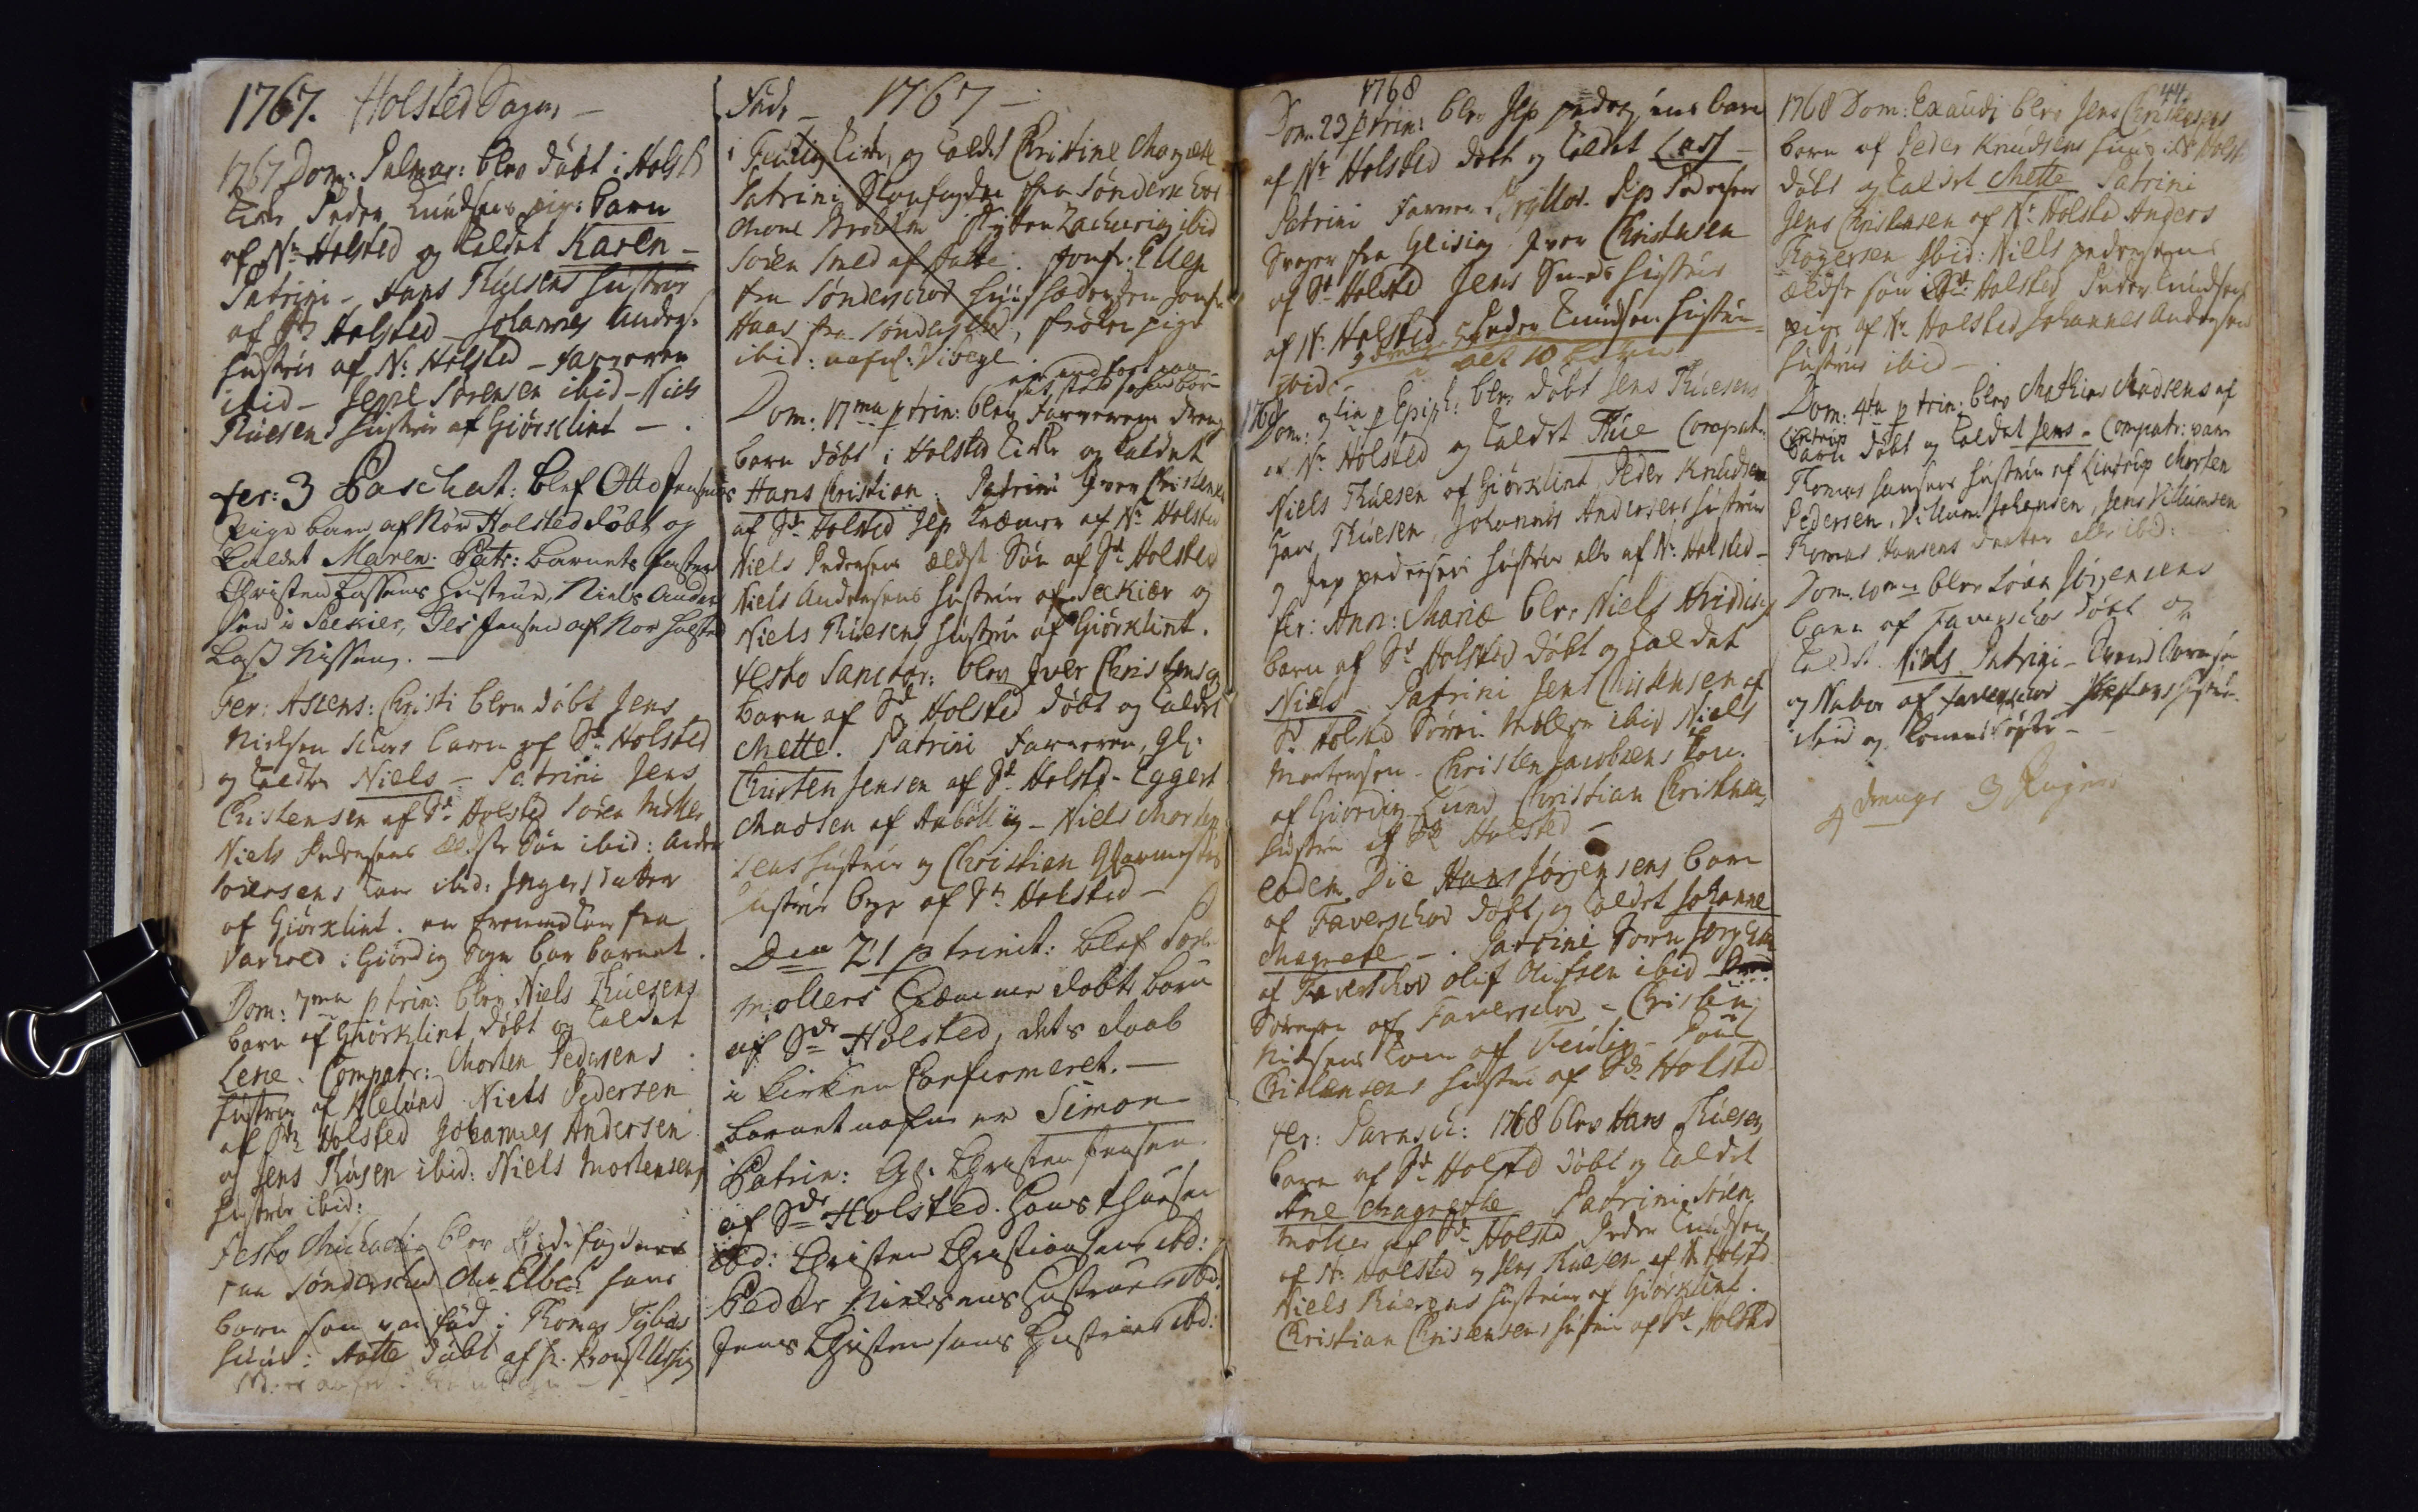1767. Holsted Sogns
1767 Dom: Palmar: blev døbt i Holsted
Kirke Peder Knudsens pige barn
af Nr Holsted og kaldet Karen -
Patrini - Hans Thuesens Hustrue
af Sdr Holsted Johannes Anders:
Hustru af N: Holsted - Farveren
ibid - Jeppe Sørrensen ibid - Niels
Tuesens Hustru af Giørklint -
Fer: 3 Paschat: blef Otto Jensens
Pige barn af Nør Holsted døbt og
kaldet Maren. Patr: Barnets Faster
Christen Lassens Hustrue, Niels Ander¬
sen i Seekier, Jes Jensen af Nor Holsted
Lass Nissen.
Fer: Ascens: Christi blev døbt Jens
Nielsen Sc##s barn af Sdr Holsted
og kaldet Niels - Patrini Jens
Christensen af Sdr Holsted Søren Møller
Niels Pedersens ældste Søn ibid: Ander
Sørensens Kone ibid. Ingers datter
af Giørklint. en fremmed Kone fra
Varhold i Giørding Sogn bar barnet.
Dom: 7ma p Trin: blev Niels Thuesens
Barn af Giørkhlint døbt og kaldet
Lene Compatr: Morten Pedersens
Hustrue af Klelund Niels Pedersen
af Sdr Holsted Johannes Andersen
og Jens Thusen ibid: Niels Mortensens
Hustrue ibid:
Festo Michaelis blev Ridefogdens
paa JSønderschov ## Elbes hans
barn som var fød i Thomas Pybas
huus i MAatte døbt af Hr. Proust Ussig
M##
1767
Føde -
i Feuling Kirke og kaldet Christine Magrete
Patrini Skoufogden fra Sønderschov
## ##roholm skytten Zacharis ibid
Søren Smed af Aatte. Jomfr Ellen
fra Sønderschov Huusholderen Jomfr
Haar fra Sønderschov, Frøkenepige
ibid: nafnl: Vibegl
## indfort paa
##et stod ## bør
Dom: 17ma p Trin: blev Faverens drenge
barn døbt i Holsted Kirke og kaldet
Hans Christian: Patrini Iver Christensen
af Sdr Holsted Jep Kræmer af Nr Holsted
Niels Pedersens ældste Søn af Sdr Holsted
Niels Andersens Hustrue af Seekier og
Niels Thuesens Hustrue af Giørklint.
Festo Sanctor: blev Iver Christensens
barn af Sdr Holsted døbt og kaldet
Mette. Patrini Farveren, gl:
Christen Jensen af Sdr Holsted Eggert
Madsen af Aabølling - Niels Morten¬
sens Hustrue og Christian Glarmesters
Hustrue bege af Sdr Holsted -
Dca 21 p Trinit: blef Søren
Møllers Hiæmme døbte barn
af Sdr Holsted, dets Daab
i Kirken Confirmeret. -
Barnet nafn er Simon
Patrin: Gl: Christen Jensen
af Sdr Holsted, Hans Thuesen
ibd: Christen Christiansens ibd:
Peder Nielsens Hustrue ibd:
Jens Christensens Hustrue ibd:
1768
Dom: 23 p Trin: blev Jep pedersens barn
af Nr Holsted det og kaldet Lass
Patrini Farver Bryllov. ##p Pedersen
Svoger fra Gliding, Iver Christensen
af Sdr Holsted, Jens Smeds Hustrue
af Nr Holsted Peder Knudsens Hustru
ibid.
5 drenge 5 piger
i alt 10 Børn.
1768
Dom: 3tia p Epiph: blev døbt Jens Thuesens
af Nr Holsted og kaldet Thue Compatr:
Niels Thuesen af Giørklint, Peder Knudsen
Hans Thuesens, Johannes Andersens Hustrue
og Jep pedersens Hustrue alle af Nr Holsted
Fer: Ann: Mariæ blev Niels Hviddings
Barn af Sdr Holsted døbt og kaldet
Niels - Patrini Jens Christensen af
Sdr Holsted Søren Møller ibid Niels
Mortensen - Christen Jacobsens Kon.
af Giording Lund Christian Christensens
Hustru af Sdr Holsted -
eodem Die Hans Jørgensens barn
af Faverschov døbt, og kaldet Johanne
Magrete Patrini Iver Jorgensen
af Faverschov Oluf Nissen ibid
Sørensen af Faverschov - Christen
Nilsens Kone af Feuling - Poul
Christensens Hustru af Sdr Holsted
Fer: Parasch: 1768 blev Hans Thuesens
barn af Sdr Holsted døbt og kaldet
Ane Magrethe Patrini Søren
Møller af Sdr Holsted, Peder Knudsen
af Nr Holsted og Jens Thuesen af Sdr Holsted
Niels Thuesens Hustrue af Giørklint
Christian Christensens Hustrue af Sdr Holsted
44.
1768 Dom: Exaudi blev Jens Christensens
Barn af Peder Knudsens Huus i Sdr Holsted
døbt og kaldet Mette Patrini
Jens Christensen af Nr Holsted Anders
Thøgersen ibid: Niels pedersens
ældste søn Sdr Holsted Peder Knudsens
pige af Nr Holsted Johannes Andersens
Hustrue ibid -
Dom: 4ta p Trin: blev Mathias Madsens af
Lintrup
barn døbt og kaldet Jens Compatr: vare
Thomas Hansens Hustrue af Lintrup Morten
Pedersen, Villum Johansen, Jens Villumsen
Thomas Hansens Daatter alle ibid:
Dom 10ma blev ## Jørgensens
barn af Faverschov døbt og
kaldet Niels Patrini Svend Sorensen
og Naboe af Faverschov ## Hustru
ibid og Konens Søster -
4 Drenge 3 Piger
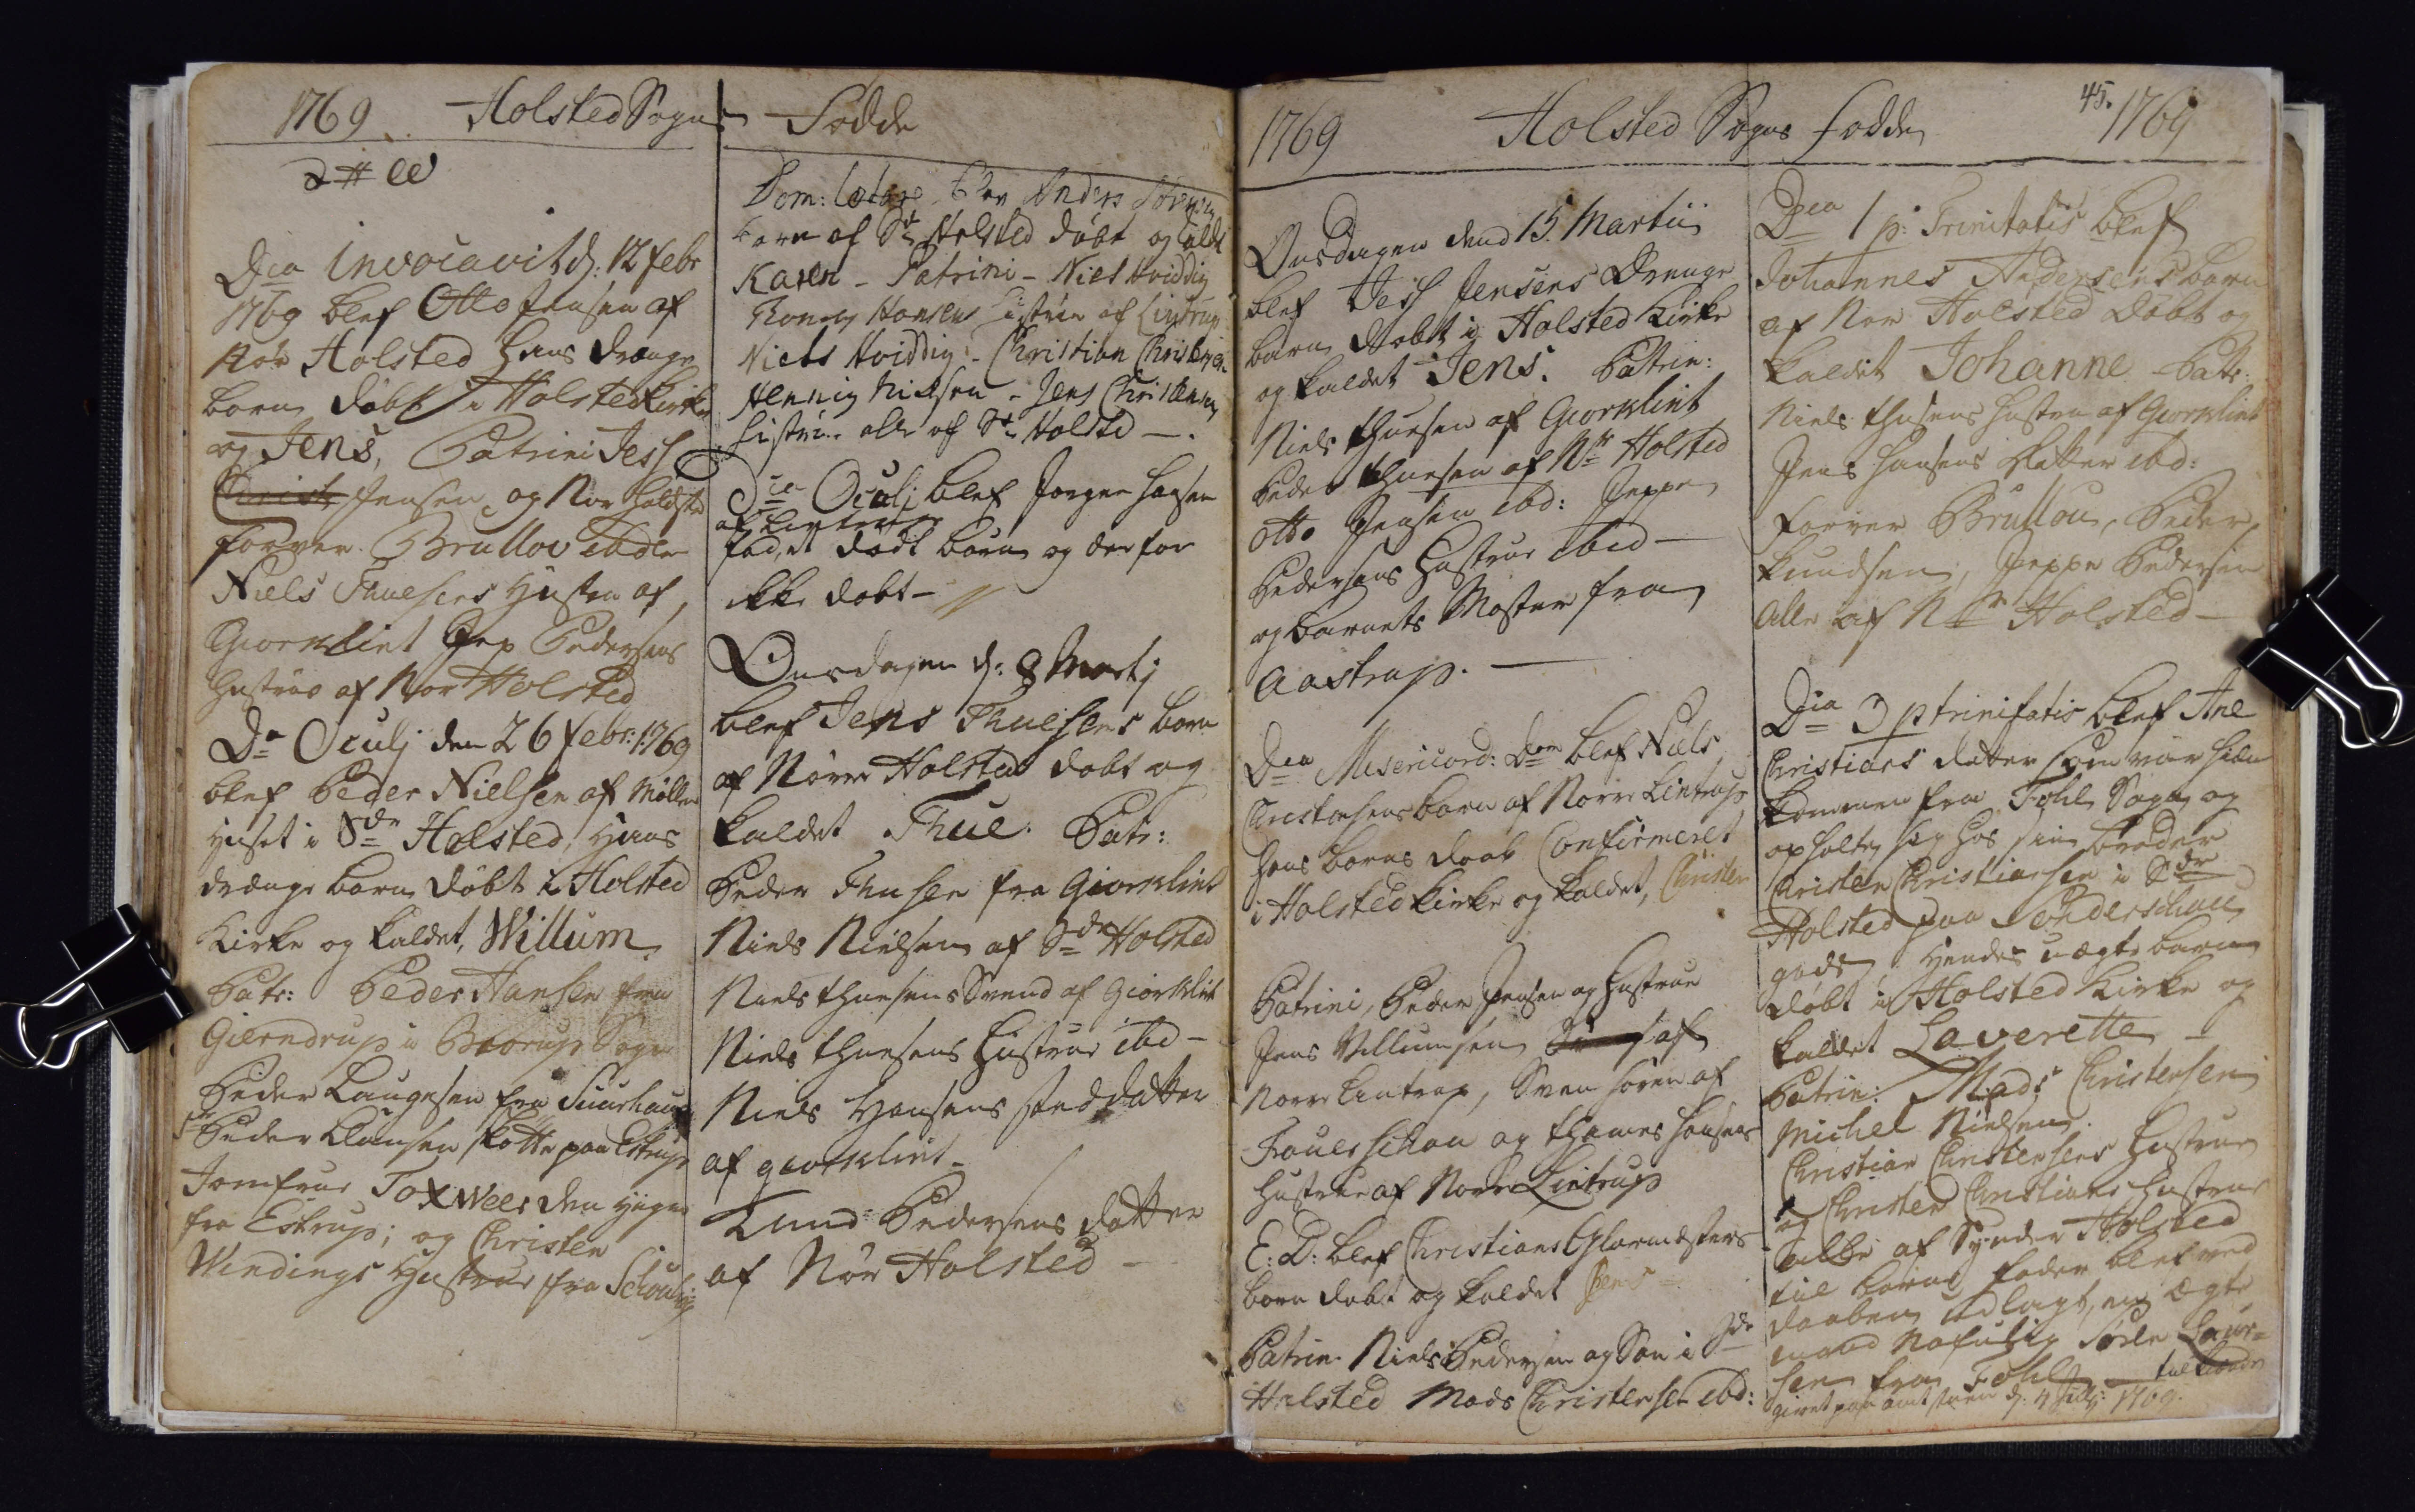Holted Sogns
1769
Dca Invocavit d: 12 Febr
1769 blef Otto Jensen af
Nør Holsted, Hans Drenge
barn døbt i Holsted Kirke
og Jens Patrini Jess
Christ Jensen og Nør Holsted
Forver Brullov ibidem
Niels Thuesens Hustru af
Giorklint Jep Pedersens
Hustrue af Nor Holsted
Dca Oculj den 26 Febr: 1769
blef Peder Nielsen af Mølle
Huuset i Sdr Holsted, Hans
drenge barn døbt i Holsted
Kirke og kaldet Willum.
Patr: Peder Hansen fra
Gierndrup i Brorup Sogn
Peder Laugesen fra Suurhauge
Peder Clausen Skøtte paa Estrup
Jomfrue Toxweer den yngre
fra Estrup; og Christen
Windings Hustrue fra Schouhaug
Fødde
Dom: Lætare blev  Anders Sørensen
barn af Sdr Holsted døbt og kaldet
Karen - Patrini - Niels Hvidding
Thomas Hansens Hustrue af Lintrup
Niels Hviddig - Christian Christensen
Hennig Nielsen - Jens Christensens
Hustrue alle af Sdr Holsted -
Dca Oculi blef Jorgen Hansen
af Lintrup
fod et dodt Barn og der for
ikke dobt -
Onsdagen d: 8 Martj
blef Jens Thuesens barn
af Nørre Holsted dobt og
kaldet Thue. Patt:
Peder Thusen fra Giørklint
Niels Nielsen af Sdr Holsted
Niels Thuesens Svend af Giørklint
Niels Thomsens Hustrue ibd -
Niels Hansens steddatter
af giorklint.
Knud Pedersens Datter
af Nør Holsted
Holsted Sogns Fodde
1769
Onsdagen dend 15. Martii
blef Jess Jensens Dreng
barn dobt i Holsted Kirke
og kaldet Jens. Patrin:
Niels Thuesen af Giorklint
Peder Thuesen af Nr Holsted
Otto Jensen ibd: Jeppe
Pedersens Hustrue ibid -
og Barnets Moster fra
Aastrup.
Dca Misericord: Dom blef Niels
Christensens Barn af Norre Lintrup
hans Barns Daab Confirmeret
i Holsted Kirke og kaldet Christen
Patrini, Peder Jensens Hustrue
Jens Villumsen S## af
Norre Lintrup, Sven soren af
Fauerschou og Thomes Hansens
Hustrue af Norre Lintrup
E: D: blef Christians Glarmæsters
barn dobt og kaldet Jens
Patrin: Niels Pedersen og Søn i Sdr
Holsted Mads Christensen ibd
45.
1769
Dca 1 p: Trinitatis blef
Johannes Andersens barn
af Nor Holsted døbt og
kaldet Johanne - Patr:
Niels Thusens Hustru af Giorklint
Jens Hansens Datter ibd:
forver Brullou, Peder
Knudsen, Jeppe Pedersen
Alle af Nr Holsted -
Dca 3 p. Trinitatis blef Ane
Christians Datter som var hiæm
kommen fra Fohl Sogn og
opholte sig hos sin broder
Christen Christiansen i Sdr
Holsted paa Sønderschous
gods, Hendes uægte barn
døbt i Holsted Kirke og
kaldet Laverette
Patrin: Mads Christensen
Michel Nielsen
Christian Christensens Hustrue
og Christen Christians Hustrue
alle af Synder Holsted
til barne fader blef ved
Daaben udlagt en Ægte¬
mand Nafnlig Søren Laur¬
sen fra Fohl - til ##
givet paa Amtstuen d: 4 Julij 1769.